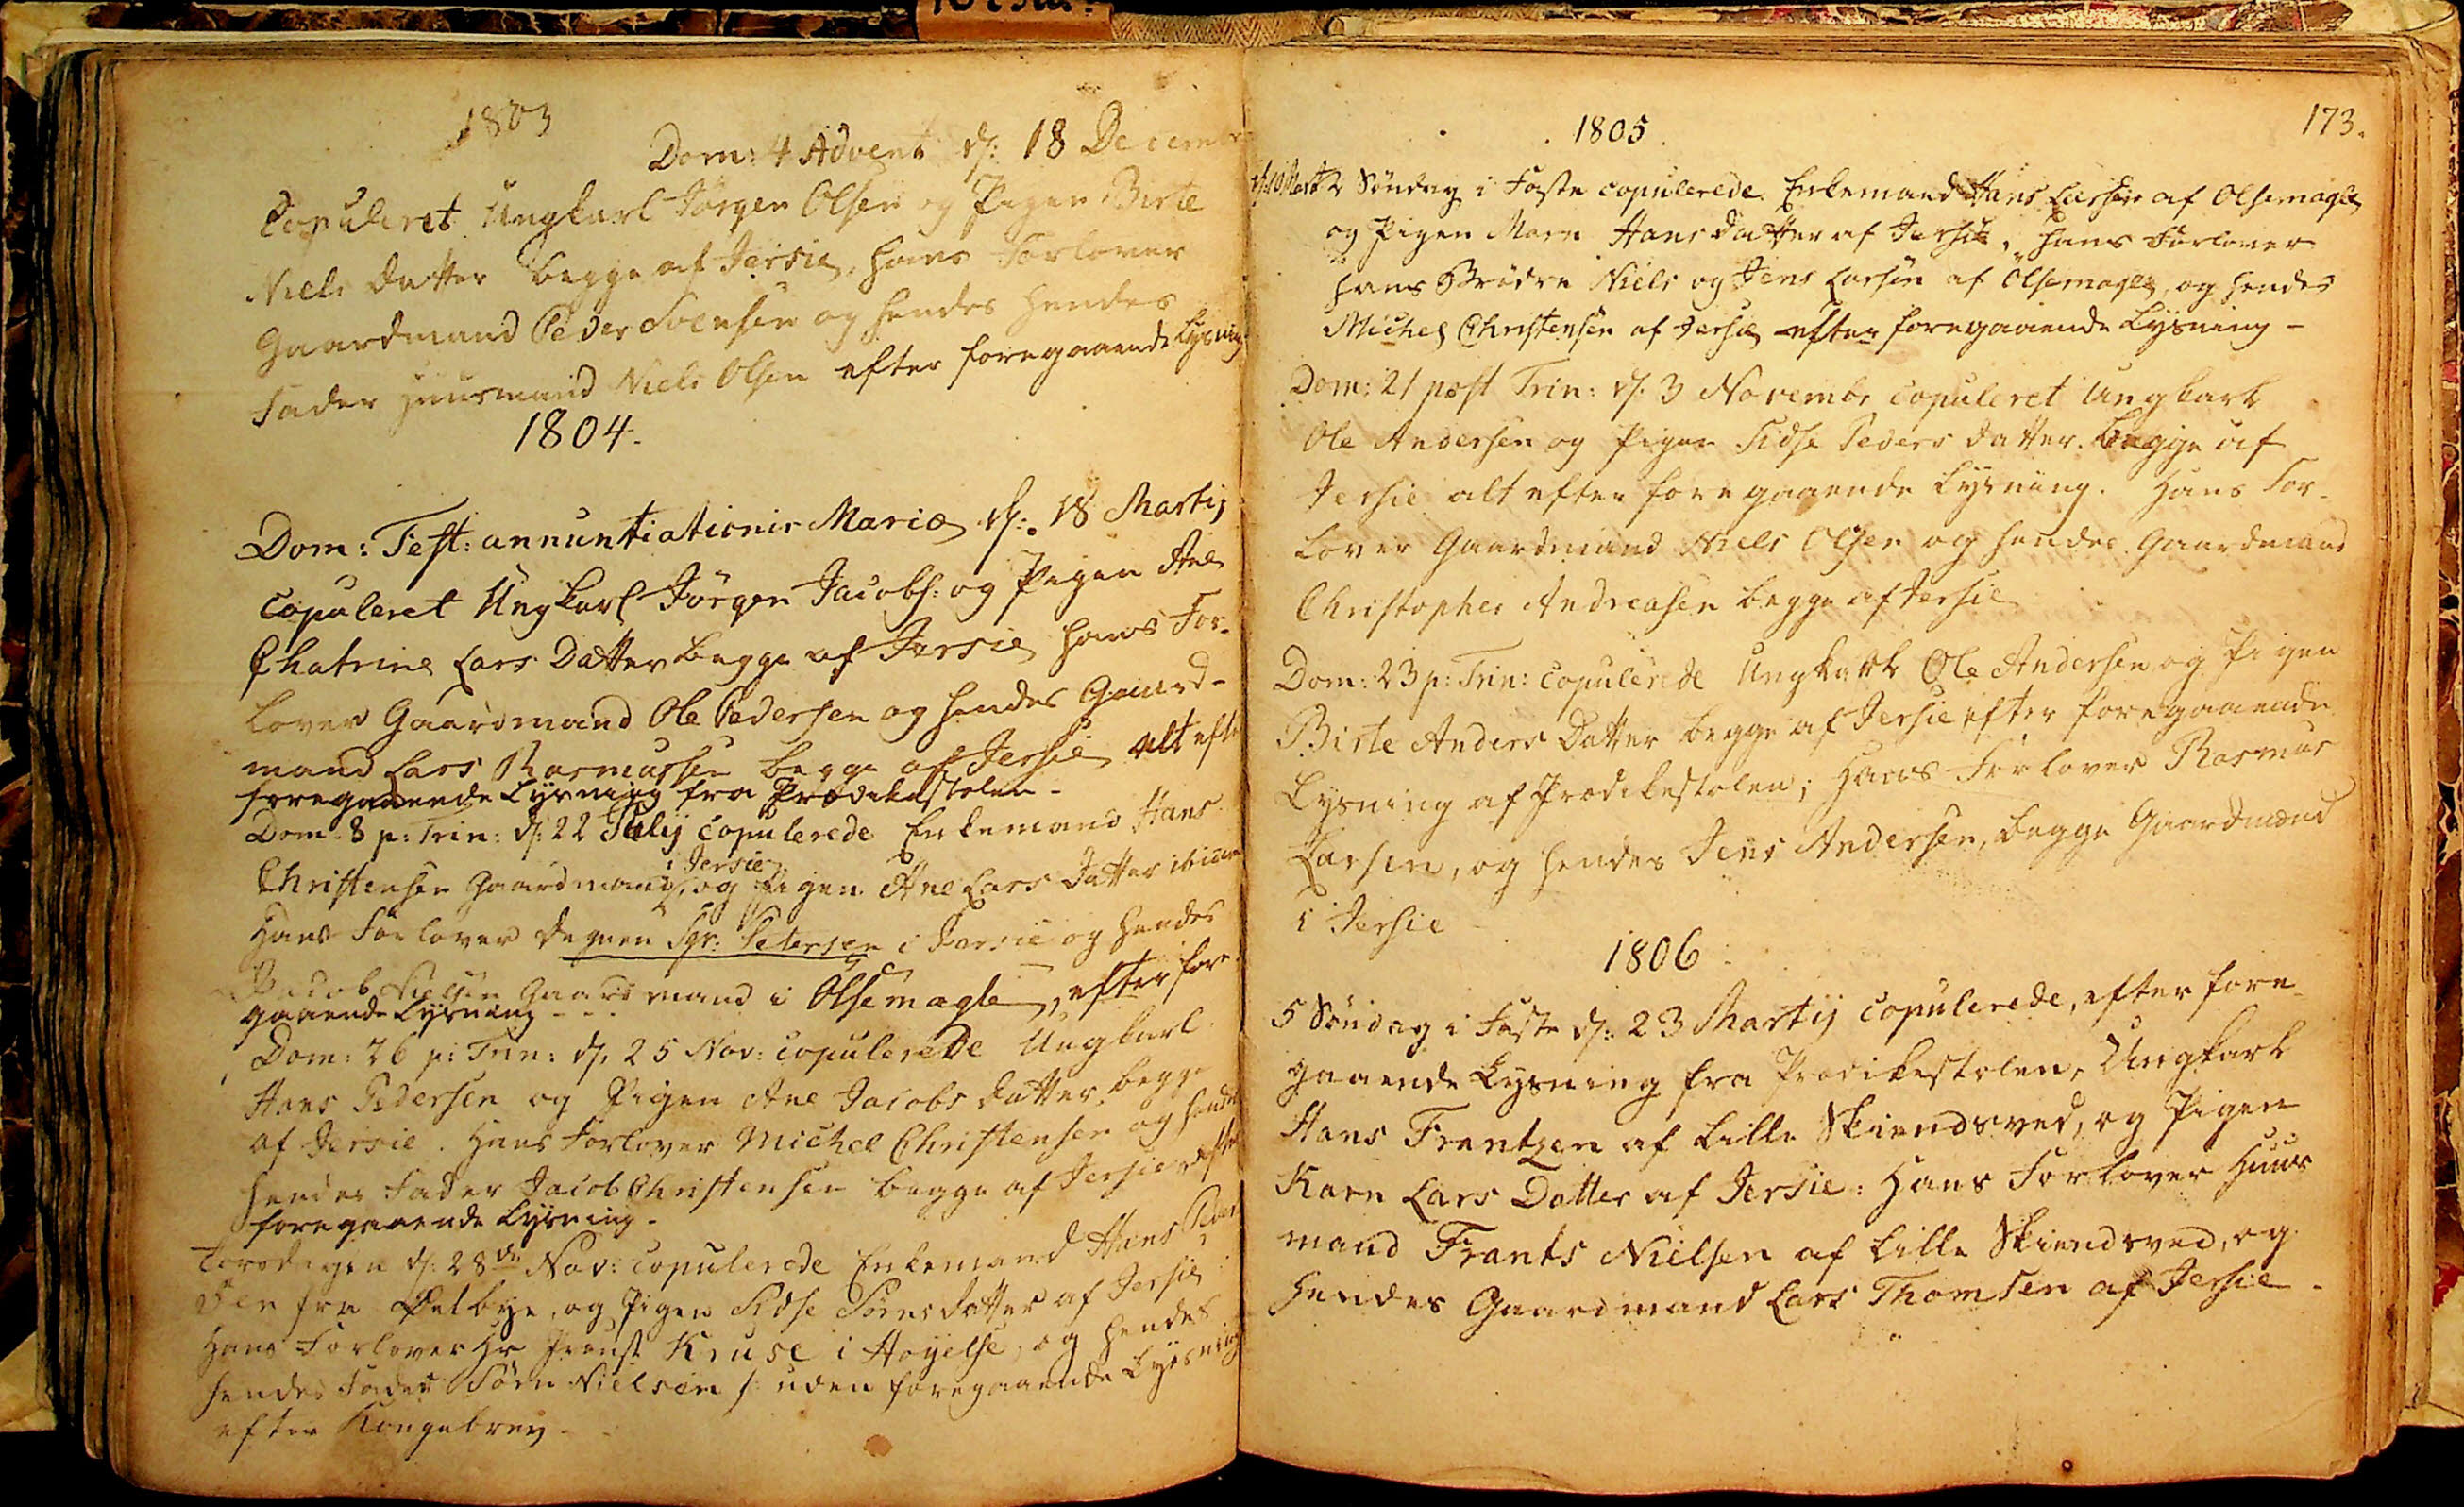1803
Dom: 4 Advent d: 18 Decembr
Copuleret Ungkarl Jørgen Olsen og Pigen Birte
Niels Datter begge af Jersie, hans Forlover
Gaardmand Peder Svensen og hendes hendes
Fader Huusmand Niels Olsen efter foregaaende Lysning
1804.
Dom: Fest: annuntiatisvir Mariæ d: 18 Martij
copuleret Ungkarl Jørgen Jacobs: og Pigen Ane
Chatrine Lars Datter begge af Jersie hans For¬
lover Gaardmand Ole Pedersen og hendes Gaard¬
mand Lars Rasmussen begge af Jersie alt efter
foregaaende Lysning fra Prædikestolen.
Dom: 8 p : Trin: d: 22 Julij copulerede Enkemand Hans
i Jersie
Christensen Gaardmand og Pigen Ane Lars Datter ibidem
Hans Forlover Degnen Sgr: Petersen i Jersie og hendes
Jacob Nielsen Gaardmand i Ølsemagle, efter fore
gaaende Lysning
Dom: 26 p: Trin: d: 25 Nov: copulerede Ungkarl
Hans Pedersen og Pigen Ane Jacobs Datter begge
af Jersie. Hans Forlover Michel Christensen og hendes
hendes Fader Jacob Christensen begge af Jersie efter
foregaaende Lysning.
Torsdagen d: 28de Nov: copulerede Enkemand Hans Peder
sen fra Dalbye, og Pigen Sidse Sørns Datter af Jersie
Hans Forlover Hr Proust Kruse i Høyelse, og hendes
hendes Fader Sørn Nielsen /: uden foregaaende Lysning
efter Kongebrev
173.
1805.
d: 10 Mart: 2 Søndag i Faste copulerede Enkemand Hans Larsen af Ølsemagle
og Pigen Marn Hans Datter af Jersie, hans Forlover
hans Brødre Niels og Jens Larsen af Ølsemagle og hendes
Michel Christensen af Jersie efter foregaaende Lysning -
Dom: 21 post Trin: d. 3 Novembr copuleret Ungkarl
Ole Andersen og Pigen Sidse Peders Datter. Begge af
Jersie alt efter foregaaende Lysning. Hans For¬
lover Gaardmand Niels Olsen og hendes Gaardmand
Christopher Andreasen begge af Jersie
Dom: 23 p: Trin: copulerede Ungkarl Ole Andersen og Pigen
Birte Anders Datter begge af Jersie efter foregaaende
Lysning af Prædikestolen; Hans Forlover Rasmus
Larsen, og hendes Jens Andersen, begge af Gaardmænd
i Jersie
1806.
5 Søndag i Faste d: 23 Martij copulerede, efter fore¬
gaaende Lysning fra Prædikestolen, Ungkarl
Hans Frantzen af Lille Skiendsved, og Pigen
Karn Lars Datter af Jersie: Hans Forlover Huus
mand Frants Nielsen af Lille Skiendsved, og
hendes Gaardmand Lars Thomsen af Jersie.
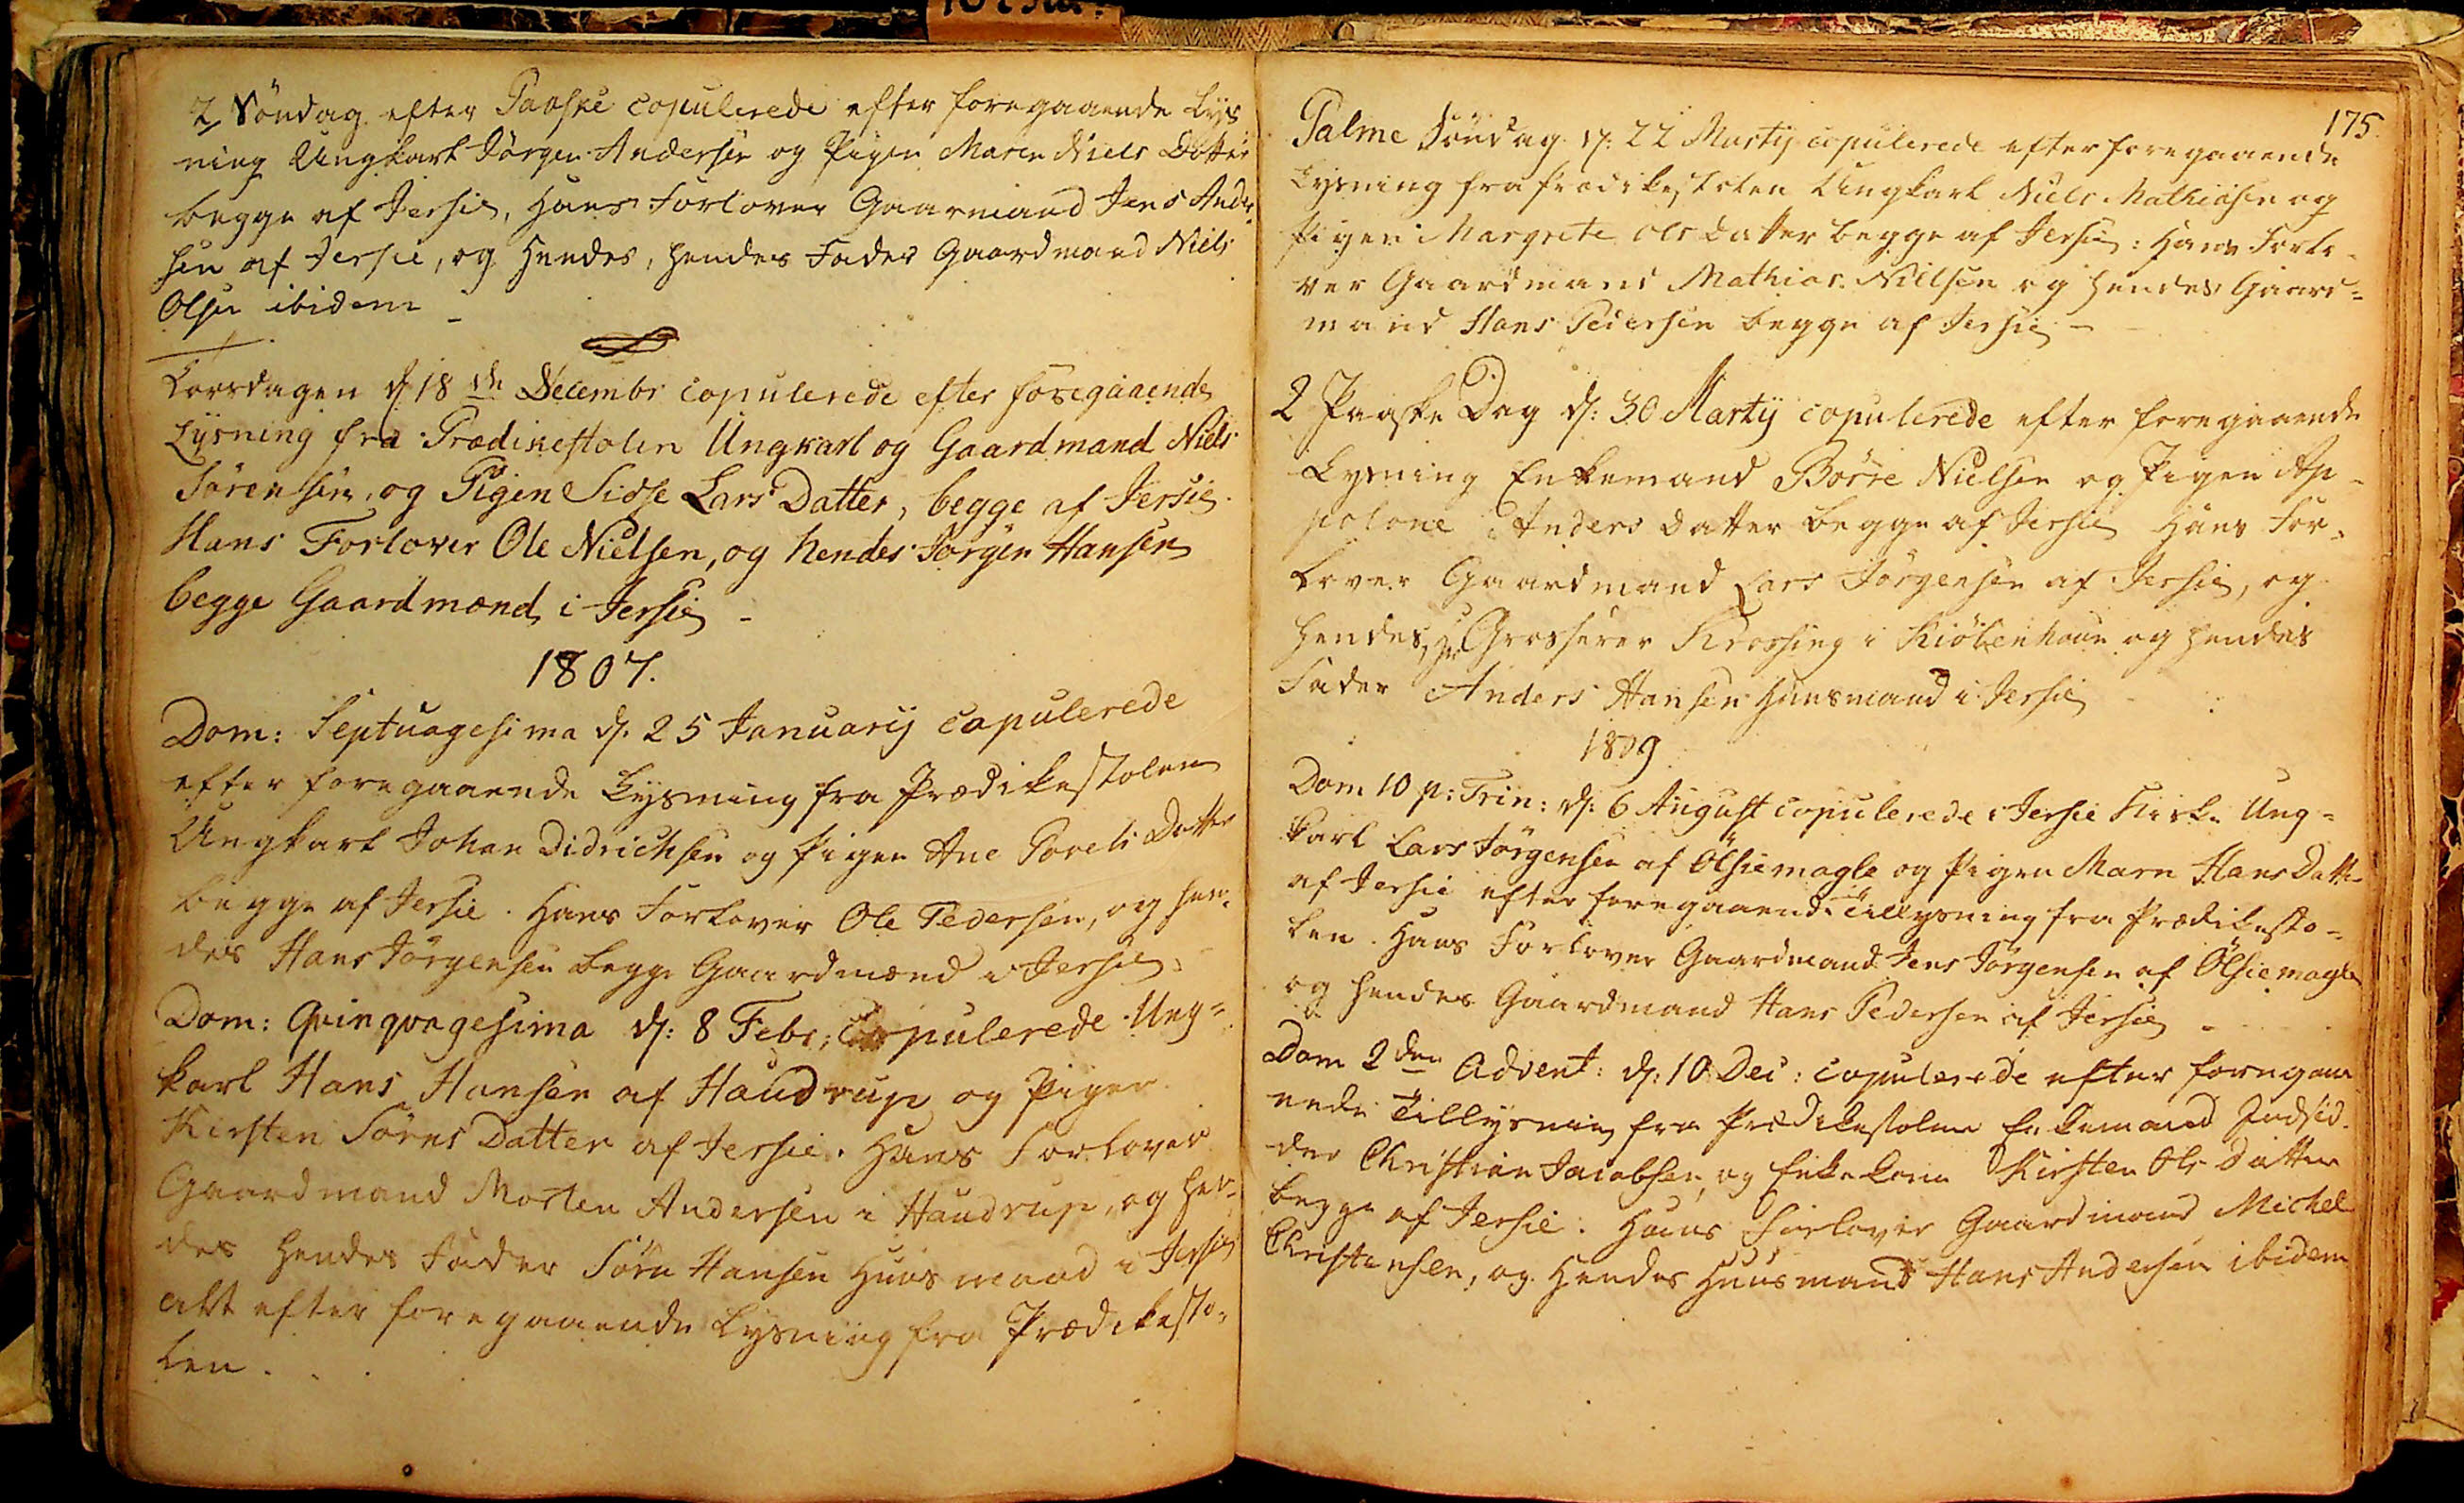2/ Søndag efter Paaske copulerede efter foregaaende Lys
ning Ungkarl Jørgen Andersen og Pigen Maren Niels Datter
begge af Jersie, Hans Forlover Gaardmand Jens Ander¬
sen af Jersie, og hendes, hendes Fader Gaardmand Niels
Olsen ibidem
Torsdagen d 18de Decembr copulerede efter foregaaende
Lysning fra Prædikestolen Ungkarl og Gaardmand Niels
Sørensen, og Pigen Sidse Lars Datter, begge af Jersie.
Hans Forlover Ole Nielsen, og hendes Jørgen Hansen
begge Gaardmænd i Jersie
1807.
Dom: Septuagesima d: 25 Januarij copulerede
efter foregaaende Lysning fra Prædikestolen
Ungkarl Johan Didrichsen og Pigen Ane Povels Datter
begge af Jersie. Hans Forlover Ole Pedersen, og hen¬
des Hans Jørgensen begge Gaardmænd i Jersie.
Dom: Qvinqvagesima d: 8 Febr: Copulerede Ung¬
karl Hans Hansen af Haudrup og Pigen
Kirsten Sørns Datter af Jersie. Hans Forlover
Gaardmand Morten Andersen i Haudrup, og hen¬
des hendes Fader Sørn Hansen Huusmand i Jersie,
alt efter foregaaende Lysning fra prædikesto¬
len 
175.
Palme Søndag d: 22 Martij copulerede efter foregaaende
Lysning fra Prædikestolen Ungkarl Niels Mathiasen og
Pigen Margrete Ols Datter begge af Jersie: Hans Forlo¬
ver Gaardmand Mathias Nielsen og hendes Gaard¬
mand Hans Pedersen begge af Jersie
2 Paaske Dag d: 30 Martij Copulerede efter foregaaende
Lysning Enkemand Børre Nielsen og Pigen Ap¬
polone Anders Datter begge af Jersie Hans For¬
lover Gaardmand Lars Jørgensen af Jersie, og
hendes, Hr Grosserer Krossing i Kiøbenhavn og hendes
Fader Anders Hansen Huusmand i Jersie
1809
Dom: 10 p: Trin: d: 6 August copulerede i Jersie Kirke Ung¬
karl Lars Jørgensen af Ølsiemagle og Pigen Marn Hans Datter
af Jersie efter foregaaende Tillysning fra Prædikesto¬
len. Hans Forlover Gaardmand Jens Jørgensen af Ølsiemagle
og hendes Gaardmand Hans Pedersen af Jersie
Dom: 2den Advent. d: 10 Dec: copulerede efter foregaa
ende Tilysning fra Prædikestolen Enkemand Indsid¬
der Christian Jacobsen, og Enkekone Kirsten Ols Datter
begge af Jersie. Hans Forlover Gaardmand Michel
Christensen, og hendes Huusmand Hans Andersen ibidem